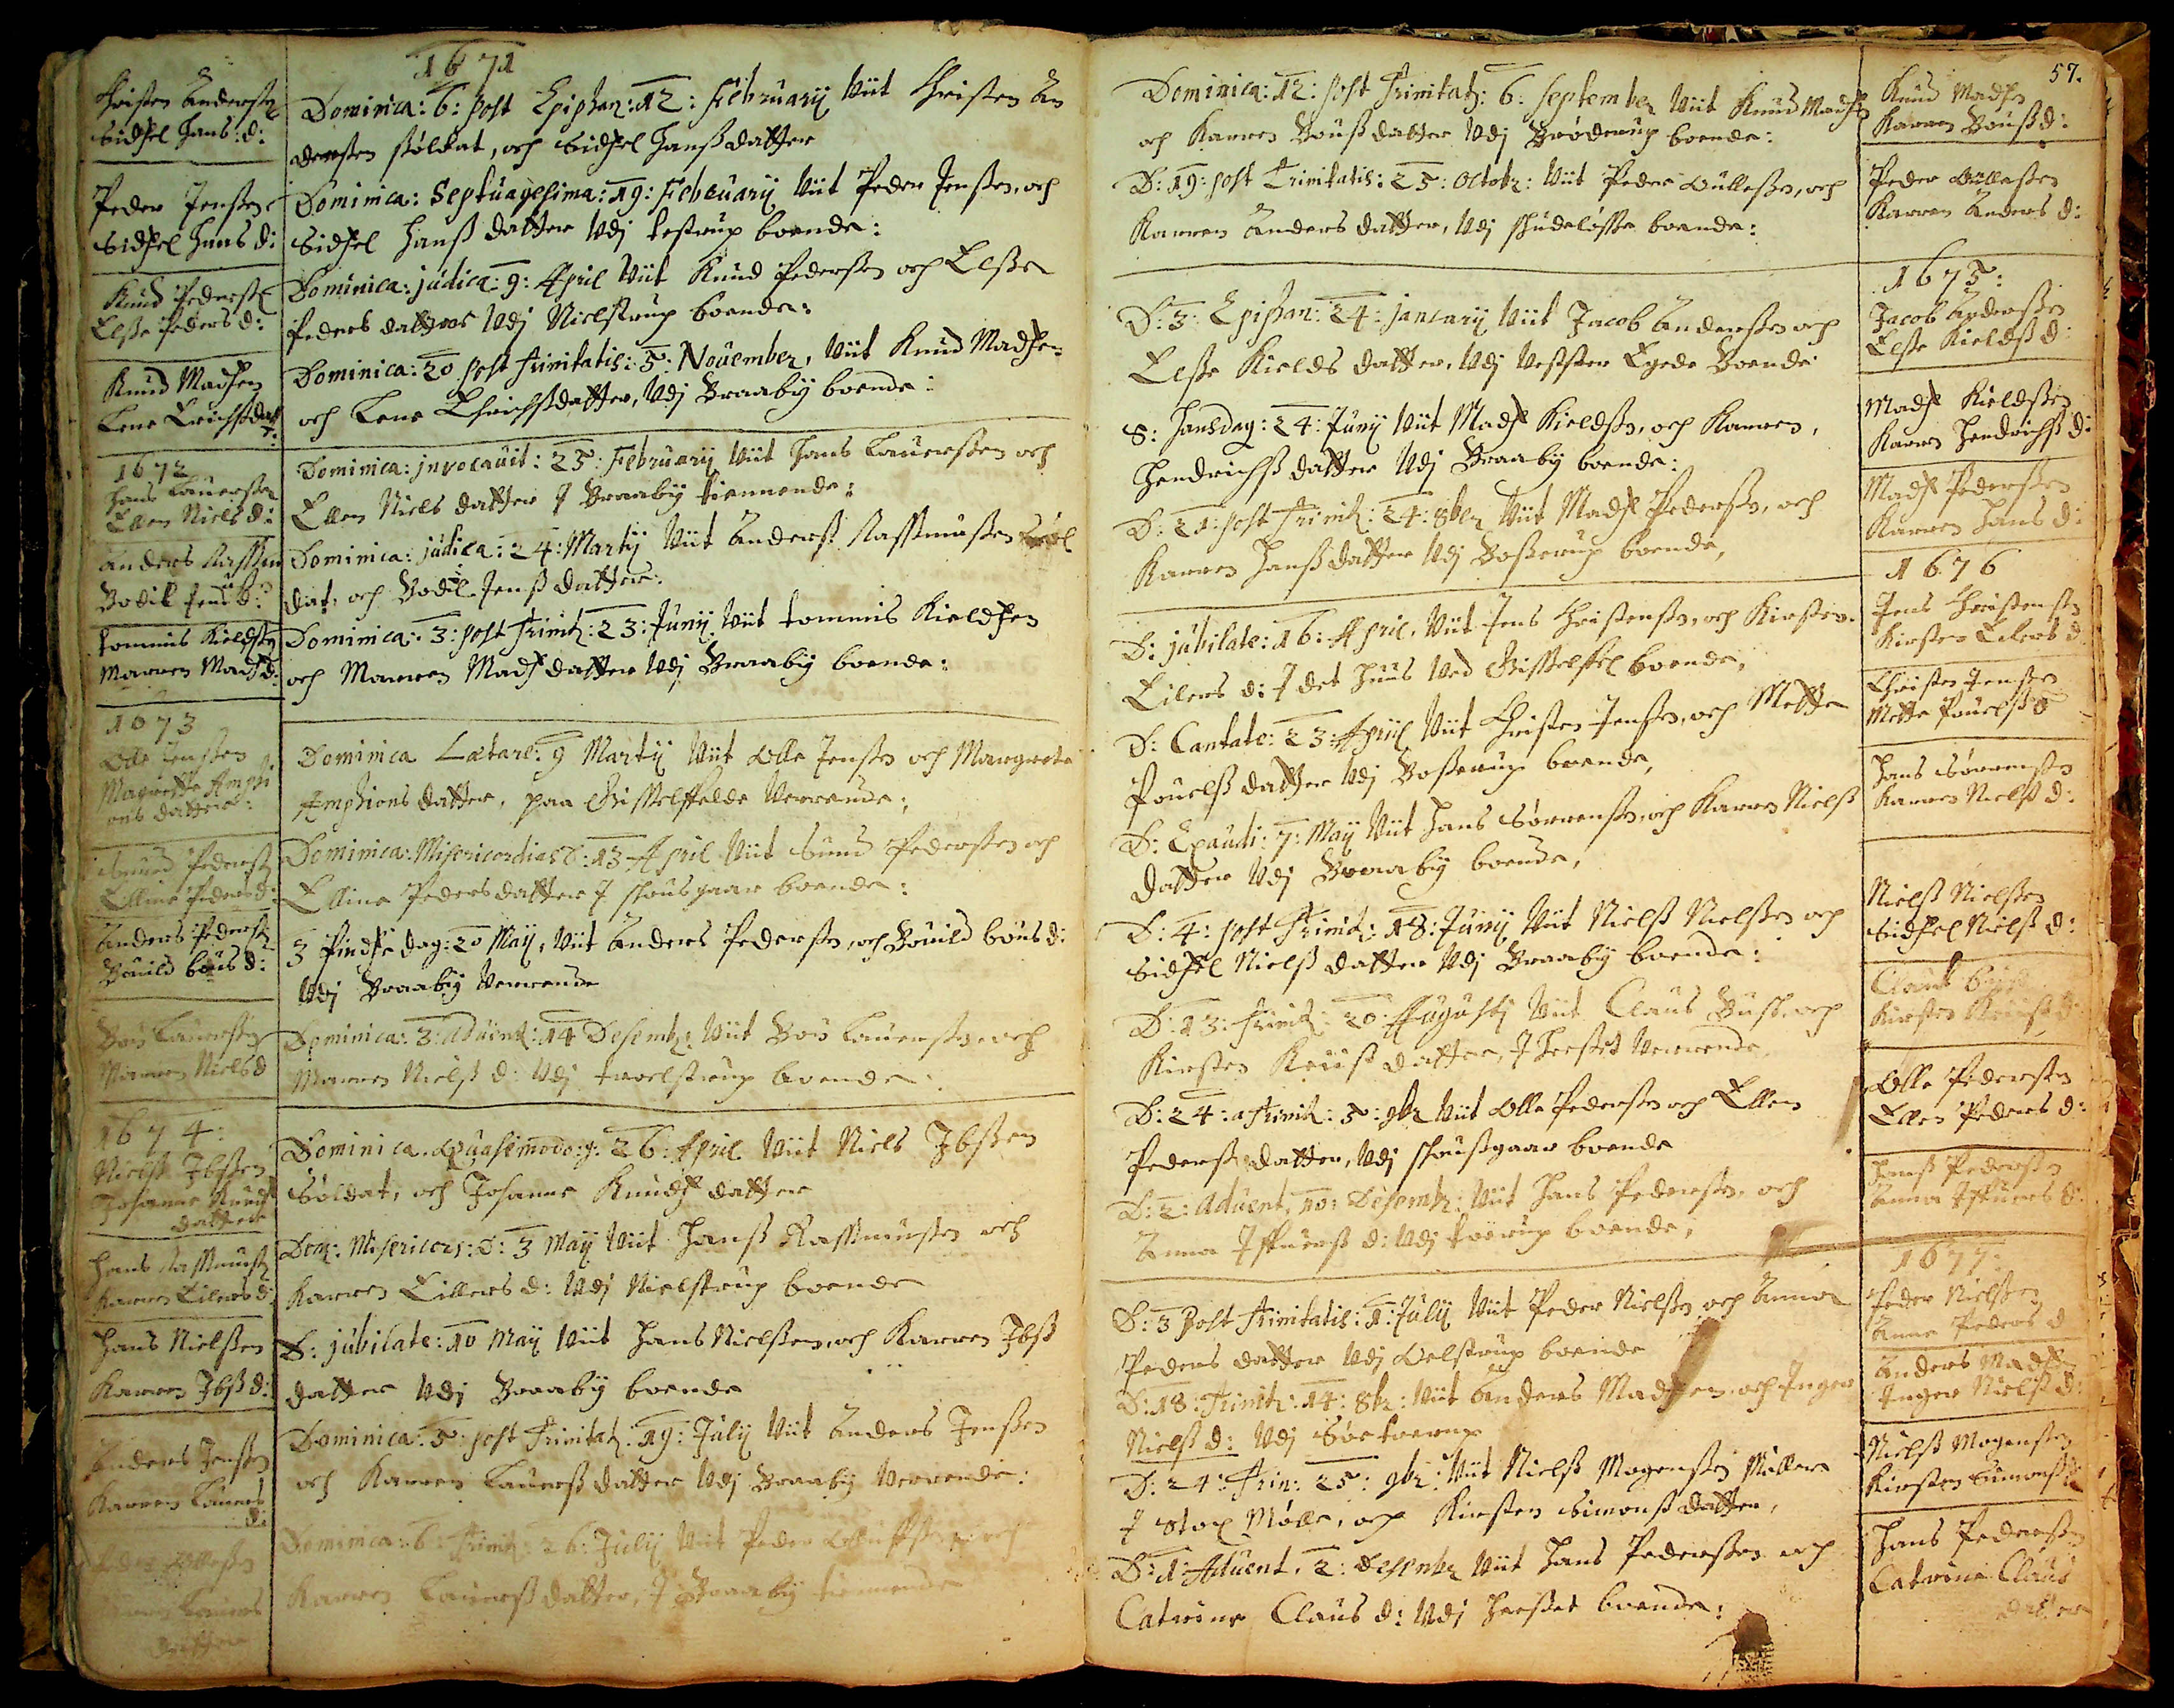Christen Andersen
Sidsel Hans:d:
1671
Dominica: 6: post Epiphan 12: February Viit Christen An
dersen Søldat, och Sidsel Hansdatter.
Peder Jensen
Sidsel Hansd:
Dominica: Septuagesima: 19: February Viit Peder Jensen, och
Sidsel Hansdatter Udj Sotorup boende:
Knud Pedersen
Else Pedersd: 
Dominica: Judica: 9: April Viit Knud Pedersen och Else
Pedersdatter Udj Nielstrup boende: 
Knud Madsøn
Lene Ehrichsdat:
Dominica: 20 post Trinitatis: 5: Nouember, Viit Knud Madsen
och Lene Ehrichsdatter, Udj Braaby boende:
1672 
Hans Lauersen
Ellen Nielsd: 
Dominica: Invocavit: 25: February Viit Hans Lauersøn och
Ellen Nielsdatter J Braaby tiennende: 
Anders Rasmus
Bodil Jensd: 
Dominica: Judica: 24: Marty Viit Anders Rasmussøn, Søl
dat, och Bodil Jensdatter:
Tommis Kieldsen
Marren Madsd:
Dominica: 3: post Trinit: 23: Juny Viit Tommis Kieldsen
och Marren Madsdatter, Udj Braaby boende:
1673
Olle Jensen
Margrete Amphi
ons datter##
Dominica Lætare: 9 Marty Viit Olle Jensen och Margrete
Amphionsdatter, paa Gisselfelde Verrende:
Suend Peders
Ellina Pedersd: 
Dominica: Misericordia: 13. April Viit Suend Pedersen och
Ellina Pedersdatter J Shousgaar boende:
Anders Pedersøn 
Bouill Bousd: 
3. Pindsedag: 20 Maij. Viit Anders Pedersøn och Bouild Bousd. 
Udj Braaby Verrende.
Bou Lauersøn 
Marren Nielsd:
Dominica: 3 Advuent: 14 Decemb: Viit Bou Lauersen och
Marren Nielsd. Udj Troelstroup boende:
1674: 
Niels Jbsen
Johanne Knuds
datter 
Dominica. Quasimodo: g: 26: April Viit Niels Jbsen
Søldat, och Johanne Knudsdatter.
Hans Rasmussen
Karren Eillersd: 
Dom: Misericordia: D: 3. May Viit Hans Rasmussen och
Karren Eillersd: Udj Nielstrup boende.
Hans Nielsen
Karren Jbsd: 
D: Jubilate: 10 May Viit Hans Nielsen och Karren Jbs
datter Udj Braaby boende
Anders Jensen
Karren Lauers
d:
Dominica: 5 post Trinitat. 19: July Viit Anders Jensen
och  Karren Lauersdatter: Udj Braaby verrende: 
Peder Ollesøn##
Karren Lauers
datter 
Dominica: 6: Trinit. 26 July Viit Peder Oluffsøn## och
Karren Lauersdatter, J Braaby tiendende. 
Dominica: 12: post Trinitat: 6. September Viit Knud Madsøn
och Karren Bousdatter Udj Brøderup boende:
57.
Knud Madsen
Karren Bousd:
D: 19: post Trinitatis: 25: Octobr: Viit Peder Oullesen, och
Karren Andersdatter, Udj Skudeløsse boende:
Peder Oullesen
Karren Andersd:
D: 3. Epiphan: 24: January Viit Jacob Andersen och
Else Kieldsdatter, Udi Vestster Egede Boende. 
1675:
Jacob Andersøn 
Else Kieldsd:
S. Hansdag: 24: Juny Viit Mads Kieldsen, och Karren
Hendrichsdatter Udj Braaby boende:
Mads Kieldsøn
Karren Henrichsd:
D: 21 post Trinit: 24. 8ber. Viit Mads Pedersen, och
Karren Hansdatter Udj Boserup boende.
Madz Pedersøn 
Karren Hansd: 
D: Jubilate: 16: April. Viit Jens Christensøn, och Kirsten
Eilersd. J det Huus Ved Gisselfel boende.
1676 
Jens Christensen
Kirsten Eilersd:
D: Cantate: 23 : Apriil Viit Christen Jensen och Mette
Pouelsdatter Udj Boserup boende,
Christen Jensen
Mette Pouelsd:
D: Exaudi. 7: May Viit Hans Sørrensen, och Karren Niels
datter Udj Braaby boende.
Hans Sørrensen
Karren Nielsd:
D: 4: post Trinit: 18: Juny Viit Niels Nielsen och
Sidsel Nielsdatter Udj Braaby boende:
Niels Nielsen
Sidsel Nielsd:
D: 13: Trinit: 20. Augusty Viit Claus Busk och
Kirsten Keiisdatter, J Hirset Verrende.
Claus Busk
Kirsten Keiisd:
D: 24: a Trinit: 5: 9br Viit Olle Pedersen och Ellen
Pedersdatter, Udj Shousgaar boende.
Olle Pedersen
Ellen Pedersd: 
D: 2: Aduent: 10: Decembr: Viit Hans Pedersen, och
Anna Jffuersd: Udj Toerup boende.
Hans Pedersen
Anna Jffuersd:
D: 3.post Trinitatis: 1 July Viit Peder Nielsøn och Anna
Pedersdatter Udj Oelstrup boende.
1677
Peder Nielsen
Anna Pedersd:
D: 18: Trinit 14: 8br: Viit Anders Madsen och Jnger
Nielsd: Udj Søetoerup.
Anders Madsen
Jnger Nielsd:
D: 24: Trin: 25: 9br: Viit Niels Mogensøn Møller 
J Stox Mølle, och Kirsten Simonsdatter.
Niels Mogensøn 
Kirsten Simonsd: 
D: 1: Aduent. 2: Decembr. Viit Hans Pedersen och
Catrine Clausd: Udj Heesed boende.
Hans Pedersøn
Catrine Claus
datter
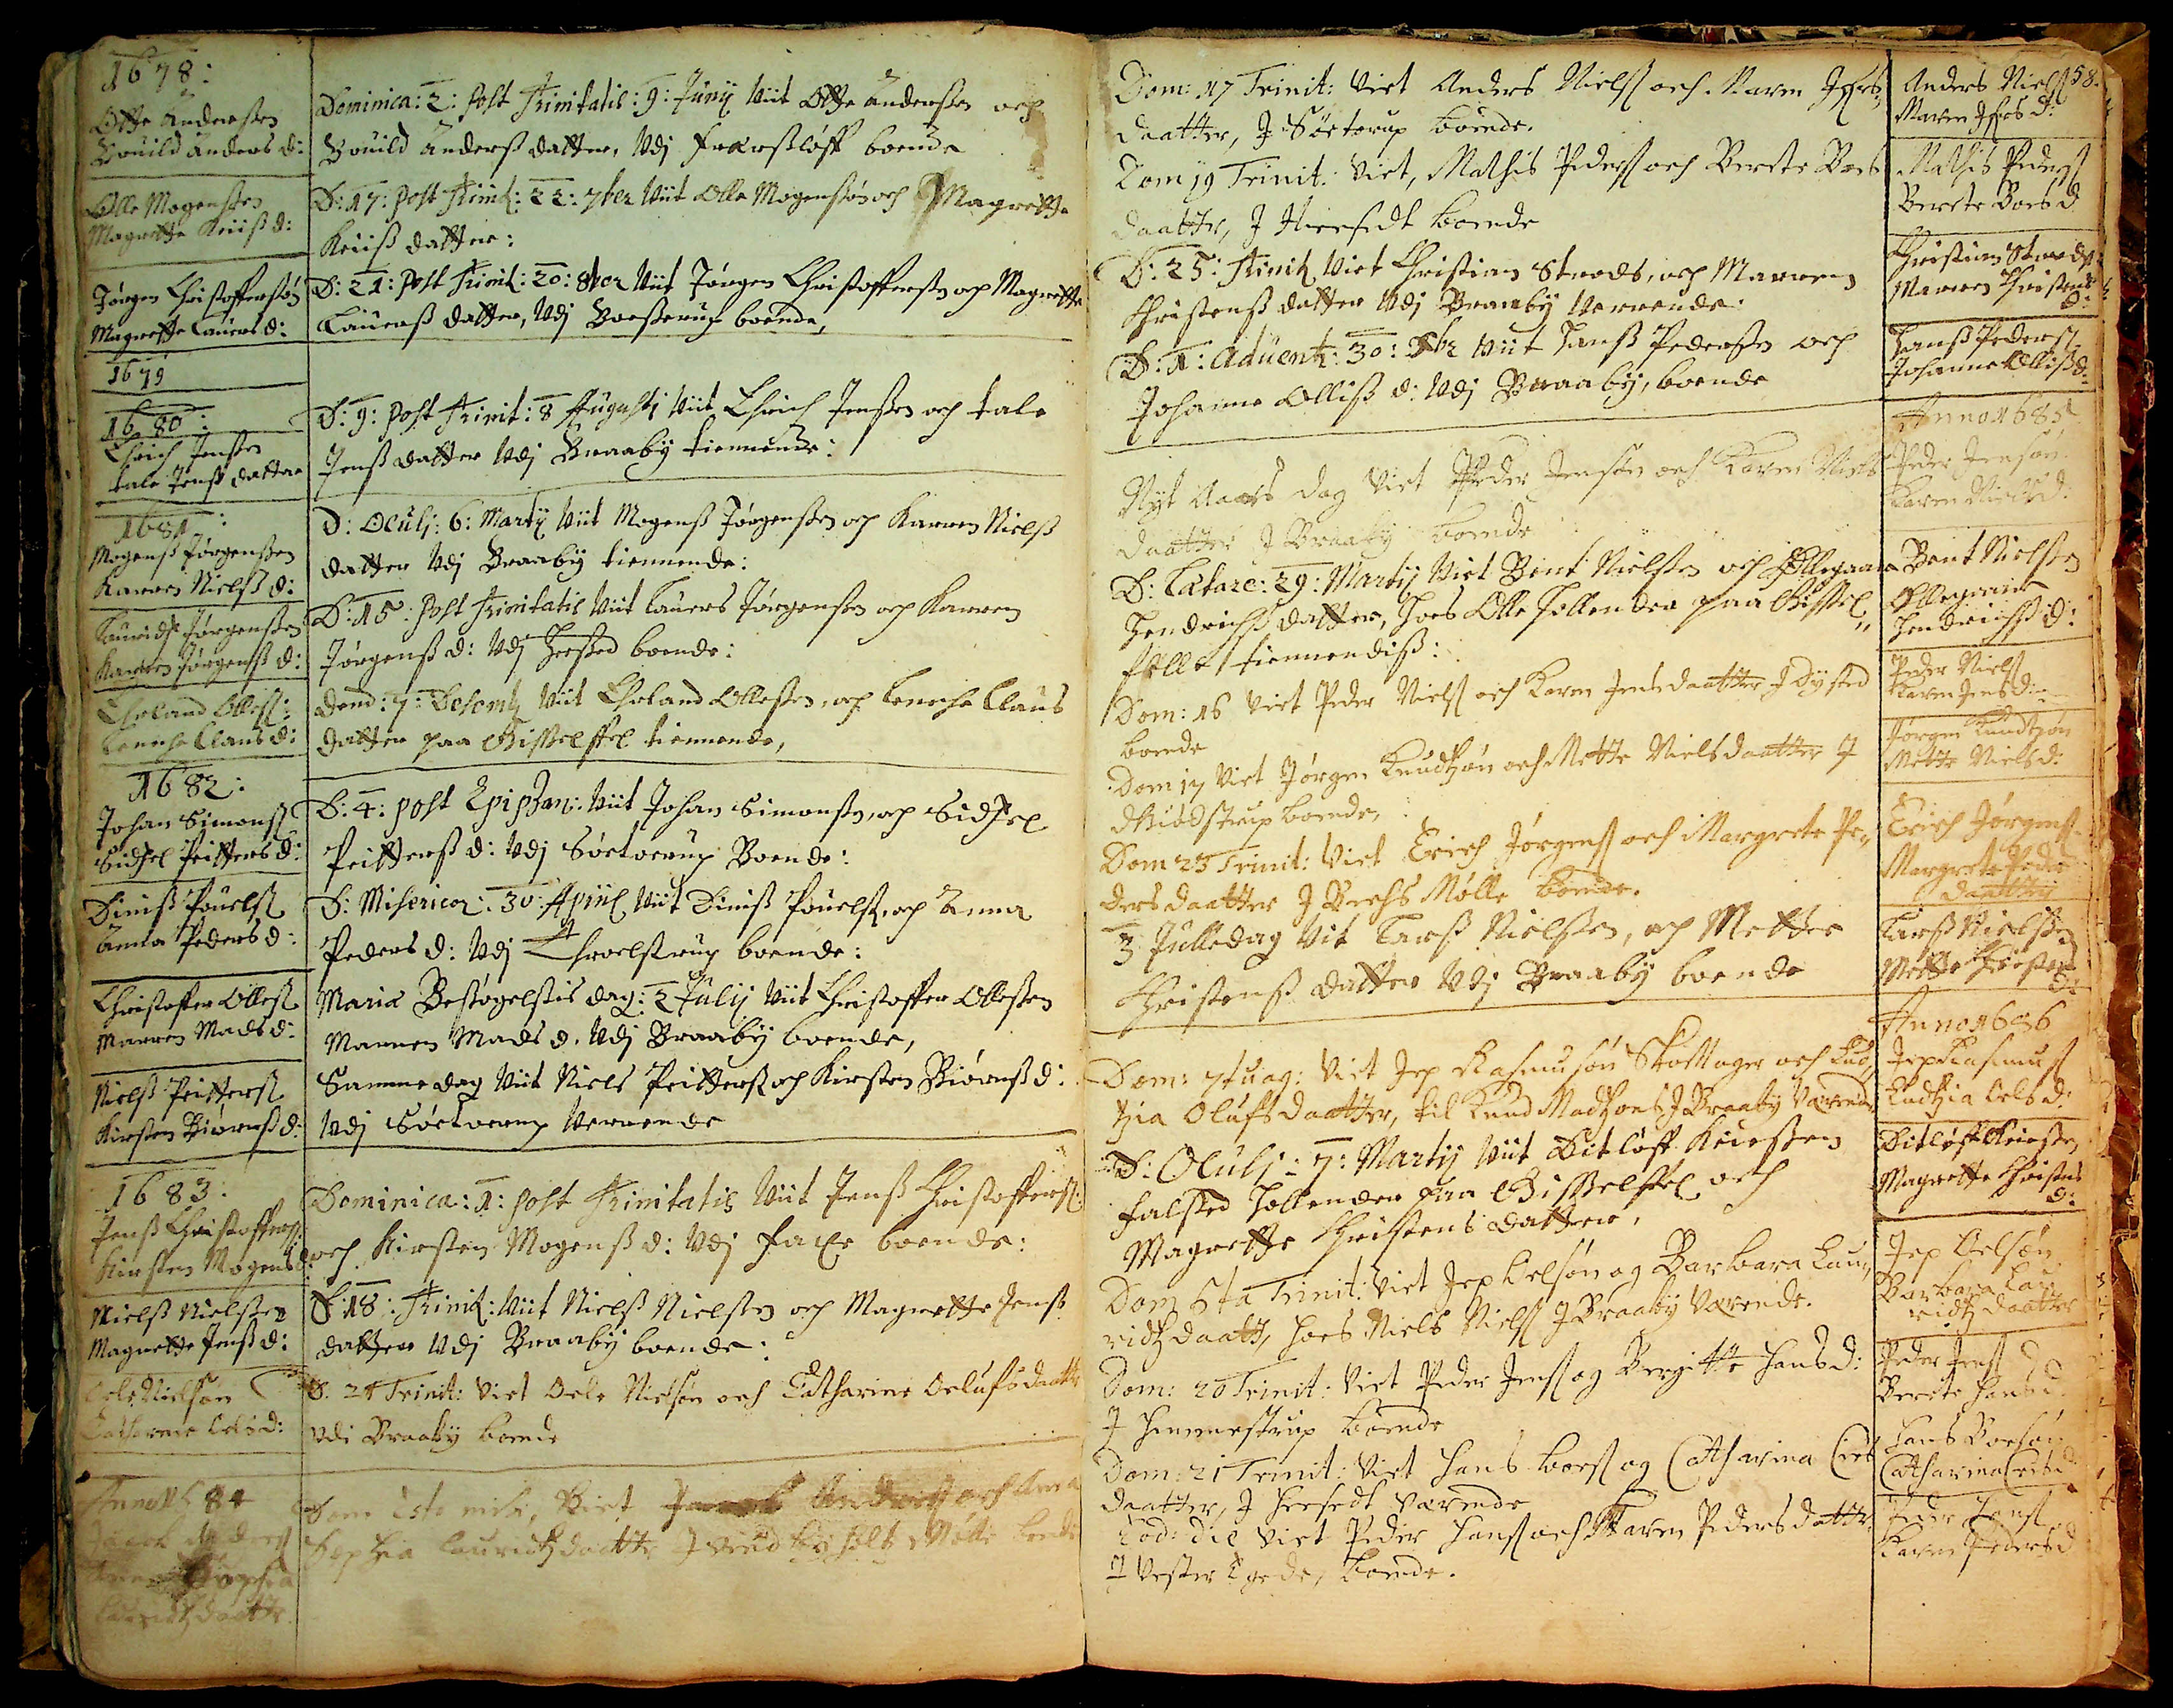1678 
Otte Andersen
Bouild Andersd: 
Dominica: 2: Post Trinitatis: 9: Juny Viit Otte Andersen och
Bouild Andersdatter, Udj Frærsløff boende.
Olle Mogensen
Margrette Kriisd:
D. 17: Post Trinit: 22: 7ber Viit Olle Mogensøn och Magrette
Kriisdatter: 
Jørgen Christoffersøn
Margrette Lauersd:
D: 21: Post Trinit: 20: 8ber Viit Jørgen Christoffersen och Magrette
Lauerssdatter, Udj Boeserup boende.
1679 
1680:
Ehrich Jensen
Tala## Jensdatter
D: 9: Post Trinit: 8 Augusti Viit Ehrich Jensen och Tala##
Jensdatter. Udj Braaby tiennende:
1681 
Mogens Jørgensen
Karren Nielsd: 
D: Oculi: 6: Marty Viit Mogens Jørgensen och Karren Niels
datter Udj Braaby tiennende:
Laurids Jørgensnn
Karren Jørgensd: 
D: 15: Post Trinitatis Viit Lauers Jørgensen och Karren
Jørgensd: Udj Hersed boende:
Ehrland Olles
Lenehe## Clausd.
Dend: 7: Desemb.Viit Ehrland Ollesen, och Lenehe## Claus
datter paa Gisselffel tiennende.
1682:
Johan Simons
Sidsel Peittersd:
D: 4: Post Epiphan: Viit Johan Simonsen, och Sidsel
Peittersd: Udj Søetoroup Boende: 
Dinis Pouels
Anna Pedersd: 
D: Misericor: 30: Apriil Viit Dinis Pouels och Anna
Pedersd: Udj Throelstrup boende:
Christoffer Olles
Marren Madsd:
Mariæ Besøgelses Dag: 2 July Viit Christoffer Ollesen,
Marren Madsd. Udj Braaby boende,
Niels Peitters
Kirsten Biørnsd:
Samme dag Viit Niels Peitters och Kirsten Biørnsd:
Udj Søetoerup Verrende.
1683
Jens Cristoffers
Kirsten Mogensd: 
Dominica: 1: Post Trinitatis Viit Jens Christoffers
och Kirsten Mogensd: Udj Faxe boende:
Niels Nielsen
Magrette Jensd:
D: 18: Trinit: Viit Niels Nielsen och Magrette Jens
datter Udj Braaby boende:
Oele Nielsøn
Catherine Oelufd:
D: 21: Trinit: Viet Oele Nielsøn och Chatharine Oelufsdatter. 
Udj Braaby boende.
Anno 1684
Jacob Fridericsøn##
Anna Sophia 
Lauridtz datter
Dom: Esto Mihi: Viet Jacob Friderics## och Anna
Sophia Lauridtzdatter, J Vindbyholt## Mølle bondis.
Dom: 17 Trinit: Viet Anders Niels och Maren Jpes
daatter, J Søetorup boende. 
58.
Anders Niels
Maren Jpesd:
Dom. 19 Trinit: Viet, Mathis Peders och Berete Boes
daatter, J Hersedt boende. 
Mathis Peders
Berete Boesd: 
D: 25: Trinit. Viet Christian Staads##, och Marren
Christensdaatter Udj Braaby Verrende.
Christian Staads##
Marren Christensd:
D: 1: Aduent: 30: Xbr. Viit Hans Pedersen och
Johanne Ollisd: Udj Braaby, boende.
Hans Peders
Johanne Ollisd:
Nyt Aars dag Viet Peder Jensøn och Karen Niels
daatter J Braaby boende.
Anno 1685 
Peder Jensøn 
Karen Nielsd:
D: Lætare: 29: Marty Viet Bent Nielsen och Øllegaard##
Hendrichsdaatter, Hoes Olle Hollænder paa Gissel¬
felldt tiennendis:
Bent Nielsøn
Øllegaard##
Hendrichsd:
Dom: 16 Viet Peder Niels och Karen Jensdaatter J Dysted
boende.
Peder Niels
Karen Jensd:
Dom 17 Viet Jørgen Knudtzøn och Mette Nielsdaatter J
Giødstrup boende. 
Jørgen Knudtzøn
Mette Nielsd:
Dom. 23 Trinit: Viet Erich Jørgens, och Margrete Pe¬
dersdaatter J Bechs Mølle boende.
Erich Jørgens
Margrete Peders¬
daatter.
3. Julledag: Viet Lars Nielsen, och Mette
Christensdaatter Udi Braaby boende.
Lars Nielsen
Mette Christensd:
Dom 7tuag Viet Jep Rasmussøn SkoMager och Lud¬
tzia Olufsdaatter, til Knud Madsøn J Braaby Værende.
Anno 1686 
Jep Rasmus
Ludtzia Oelsd:
D: Oculi: 7: Marty Viit Ditløft Kiusten##
Falsted Hollænder paa Gisselfel och
Margrette Christensdatter.
Ditløft Kriestsøn##
Margrette Christens
d: 
Dom: 6ta Trinit: Viet Jep Oelsøn og Barbara Lau¬
ridtzdaatter, hoes Niels Niels J Braaby Værende.
Jep Oelsøn
Barbara Lau
ridtzdaatter
Dom: 20 Trinit: Viet Peder Jens og Birgitte Hansd:
J Himmenstrup boende.
Peder Jens
Berete Hansd:
Dom: 21 Trinit: Viet Hans Boes og Catharina Erech##
daatter, J Hersedt værende.
Hans Boesøn
Catharina Erechd##
Eod: die Viet Peder Hans och Karen Pedersdatter
J Vester Egede boende. 
Peder Hans
Karen Pedersd: